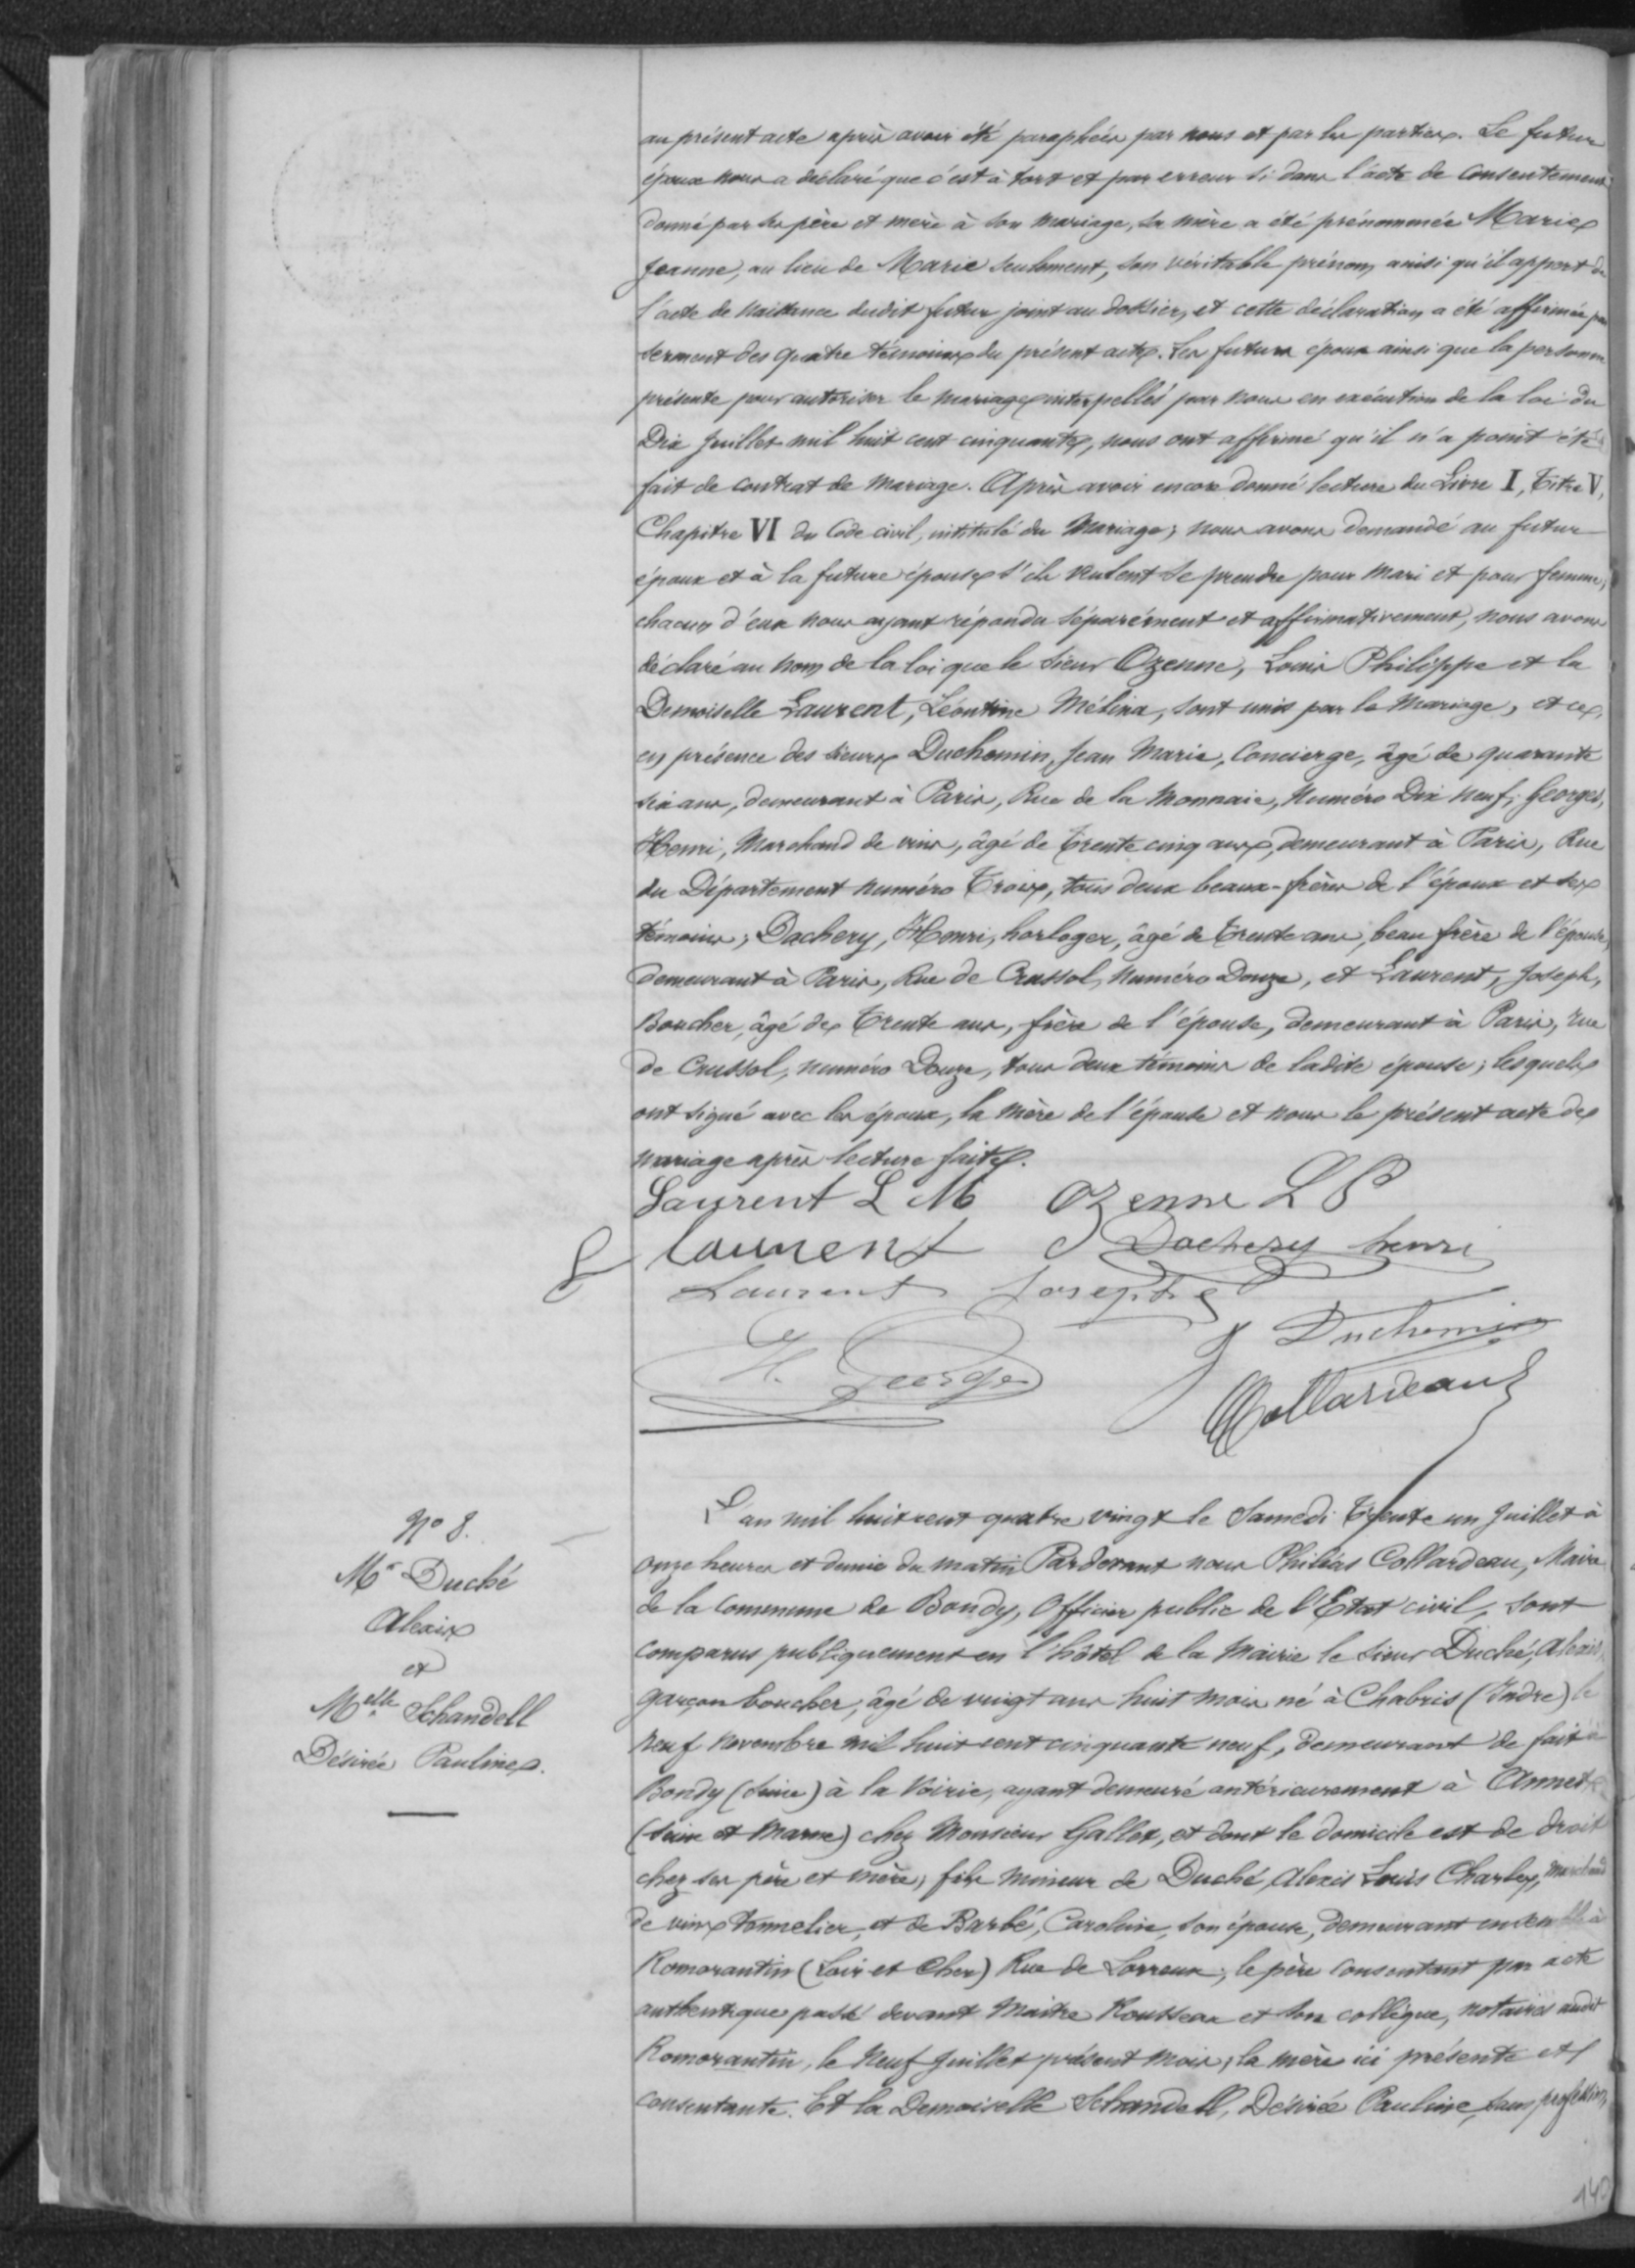au présent acte après avoir été paraphées par nous et par les parties. Le futur
époux nous a déclaré que c'est à tort et par erreur si dans l'acte de consentement
donné par les père et mère à son mariage, sa mère a été prénommée Marie
Jeanne, au lieu de Marie seulement, son véritable prénom ainsi qu'il apport de
l'acte de naissance dudit futur joint au dossier, et cette déclaration a été affirmée par
sement des quatre témoins du présent acte. Les futurs époux ainsi que la personne
présente pour autoriser le mariage interpellés par nous en exécution de la loi du
Dix Juillet mil huit cent cinquante, nous ont affirmé qu'il n'a point été
fait de contrat de mariage. Après avoir encore donné lecture du Livre I, Titre V,
Chapitre VI du Code civil, intitulé du Mariage, nous avons demandé au futur
époux et à la future épouse s'ils veulent se prendre pour mai et pour femme
chacun d'eux nous ayant répondu séparément et affirmativement, nous avons
déclaré au nom de la loi que le sieur Ozenne, Louis Philippe et la
Demoiselle Laurent, Léontine Mélina, sont unis par le mariage, et ce
en présence des sieurs Duchemin Jean Marie, concierge âgé de quarante
six ans, demeurant à Paris, Rue de la monnaie, numéro Dix neuf; Georges
Henri; marchand de vins, âgé de trente cinq ans, demeurant à Paris, Rue
du Département numéro Trois, tous deux beaux-frère de l'époux et des
témoins, Dachey, Henri, horloger, âgé de trente ans, beau frère de l'épouse
demeurant à Paris, Rue de Crassol, Numéro douze, et Laurent, Joseph,
Boucher, âgé de trente ans, frère de l'épouse, demeurant à Paris, rue
de Crussol, numéro Douze, tous deux témoins de ladite épouse; lesquels
ont signé avec les époux, la mère de l'épouse et nous le présente acte des
mariage après lecture faite
N°8
M Duché
Aleain
et
Melle Schandell
Désirée Pauline
L'an mil huit cent quatre vingt le Samedi Trente un Juillet à
onze heures et demie du matin Pardevant nous Philias Collardeau, Maire
de la Commune de Bondy, Officier public de l'Etat civil, sont
comparus, publiquement en l'hôtel de la Mairie le sieur Duché, Aleain
garçon boucher, âgé de vingt ans huit mai né à Chabris (Indre) le
neuf novembre mil huit cent cinquante neuf, demeurant de fait à
Bondy (Seine) à la Voirie, ayant demeuré antérieurement à Annet
(Seine et Marne) chez Monsieur Gallet, et dont le domicile est de droit
chez ses père et mère, fils messieur de Duché, alexis Louis Charley, marchand
de vins tomelier, et de Barbé, Caroline, son épouse, demeurant ensemble à
Romarantin (Loir et Cher) Rue de Lorreux; le père consentant par acte
authentique passé devant Maire Rousseau et son collègue, notaire audit
Romarantin, le neuf Juillet présent mois, la mère ici présente et
consentante Et la Demoiselle Sehandell Désirée Pauline, sans profession
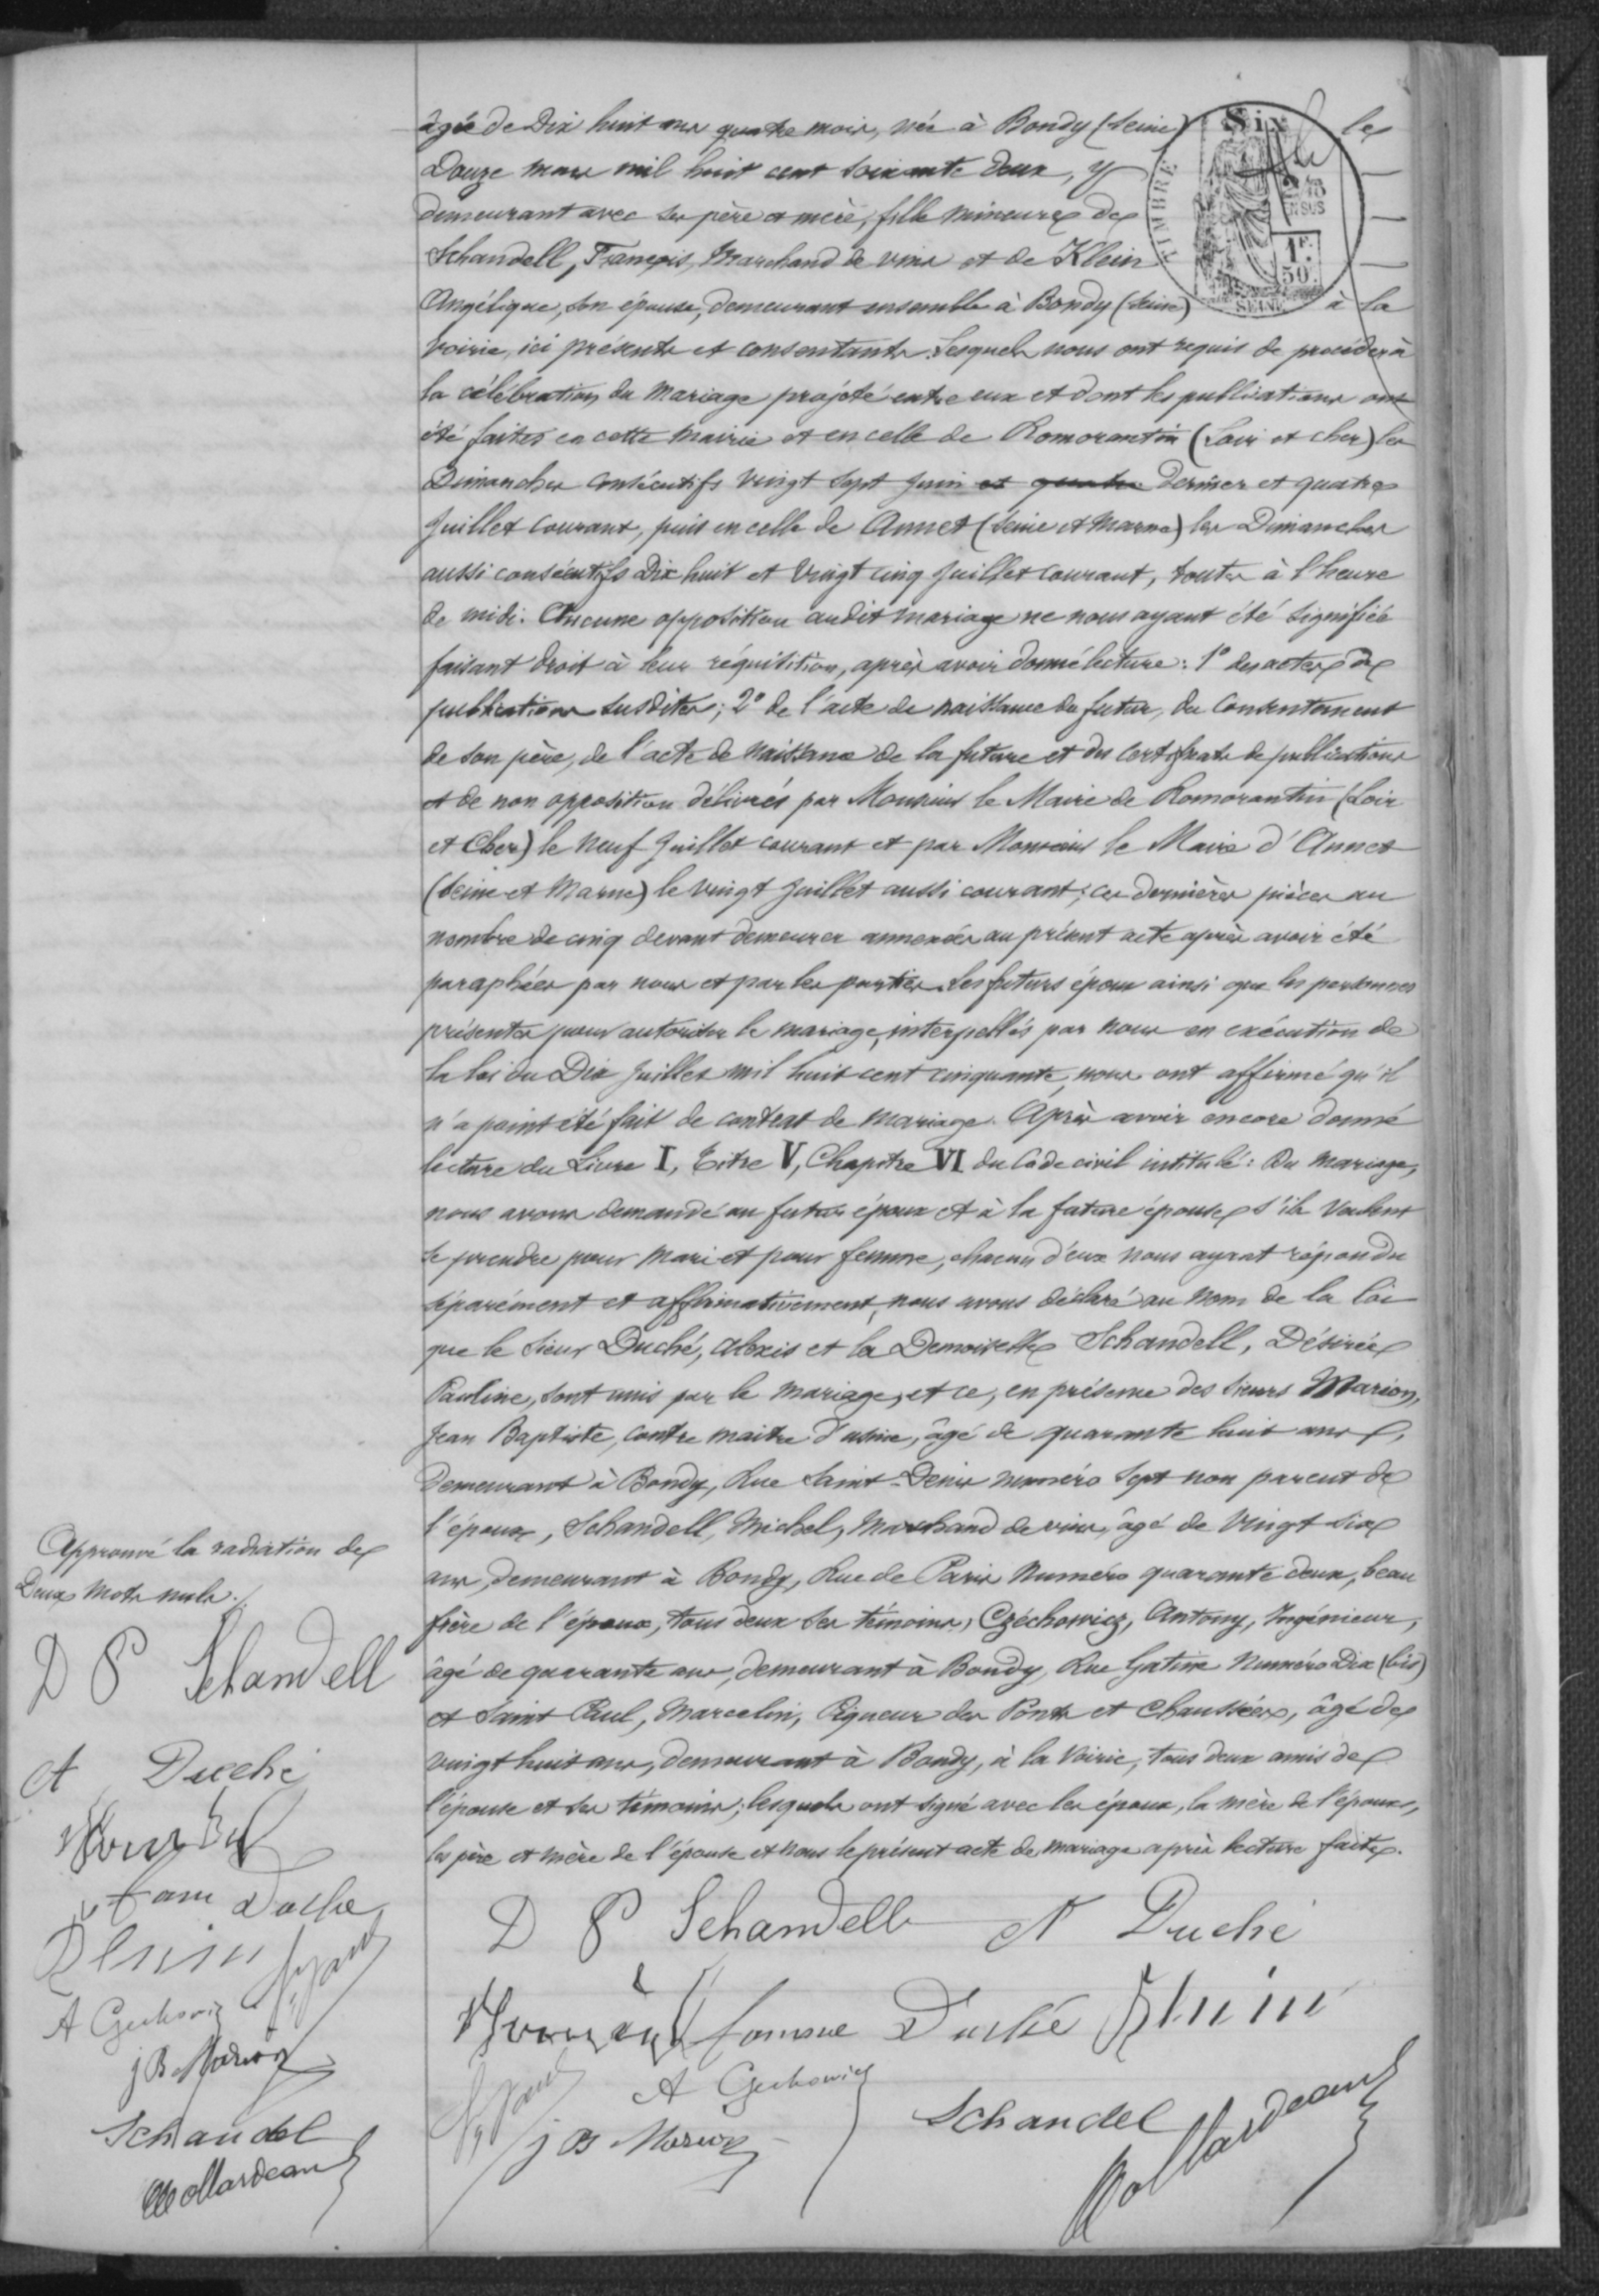âgée de dix huit ans quatre mois, née à Bondy (Seine)
Douze mars mil huit cent soixante deux, y
demeurant avec ses père et mère, fille mineure des
Schandelle, François, marchand de vins et de Klein
Angélique, son épouse, demeurant ensemble à Bondy (Seine) à la
voirie, ici présente et consentante, Lesquels nous ont requis de procéder à
la célébration de mariage projeté entre eux et dont les publications ont
été faites en cette mairie et en celle de Romorantin (Loir et Cher) les
Dimanches consécutifs vingt sept juin et quatre dernier et quatres
Juillet courant, puis en celle de Annet (Seine et Marne) les Dimanches
aussi consécutifs Dix huit et vingt cinq Juillet courant, toute à l'heure
de midi: Aucune opposition audit mariage de nous ayant été signifiée
faisant droit à leur réquisition, après avoir donné lecture: 1° des actes de
publications susdites; 2° de l'acte de naissance du futur, du consentement
de son père, de l'acte de naissance de la future et du certificat de publication
et de non opposition délivrés par Monsieur le Maire de Romorantin (Loir
et Cher) le neuf Juillet courant et par Monsieur le Maire d'Annet
(Seine et Marne) le vingt juillet aussi courant; ces dernières pièces au
nombre de cinq devant demeurer annexées au présent acte après avoir été
paraphées par nous et pas les parties. Les futures époux ainsi que les personnes
présentes pour autoriser le mariage, interpellés par nous en exécution de
la loi du Dix juillet mil huit cent cinquante, nous ont affirmé qu'il
n'a point été fait de contrat de mariage. Après avoir encore donné
lecture du Livre I, Titre V, Chapitre VI du Code civil intitulé: Du mariage,
nous avons demandé au futur époux et à la future épouse s'ils veulent
se pendre pour mari et pour femme, chacun d'eux nous ayant répondu
séparément et affirmativement, nous avons déclaré au nom de la loi
que le sieur Duché, Alexis et la Demoiselle Schandelle, Désirée
Pauline, sont unis par le mariage, et ce, en présence des sieurs Marion,
Jean Baptiste, contre maitre d'autre, âgé de quarante huit ans l'
demeurant à Bondy, Rue Saint-Denis, numéro sept non parent de
l'époux, Schandell, Michel, marchand de vin, âgé de vingt six
ans, demeurant à Bondy, Rue de Paris numéro quarante deux, beau
frère de l'époux, tous deux des témoins, Czéchannicz, Antoine, Impérieur,
âgé de quarante ans, demeurant à Bondy, Rue Gatine Numéro Dix (bis)
et Saint Paul, Marcelin, Piqueur des Ponts et Chaussées, âgée de
vingt huit ans, demeurant à Bondy, à la voirie, tous deux amis de
l'époux et des témoins; lesquels ont signé avec les époux, la mère de l'époux,
les père et mère de l'épouse et nous le présent acte de mariage après lecture faite.